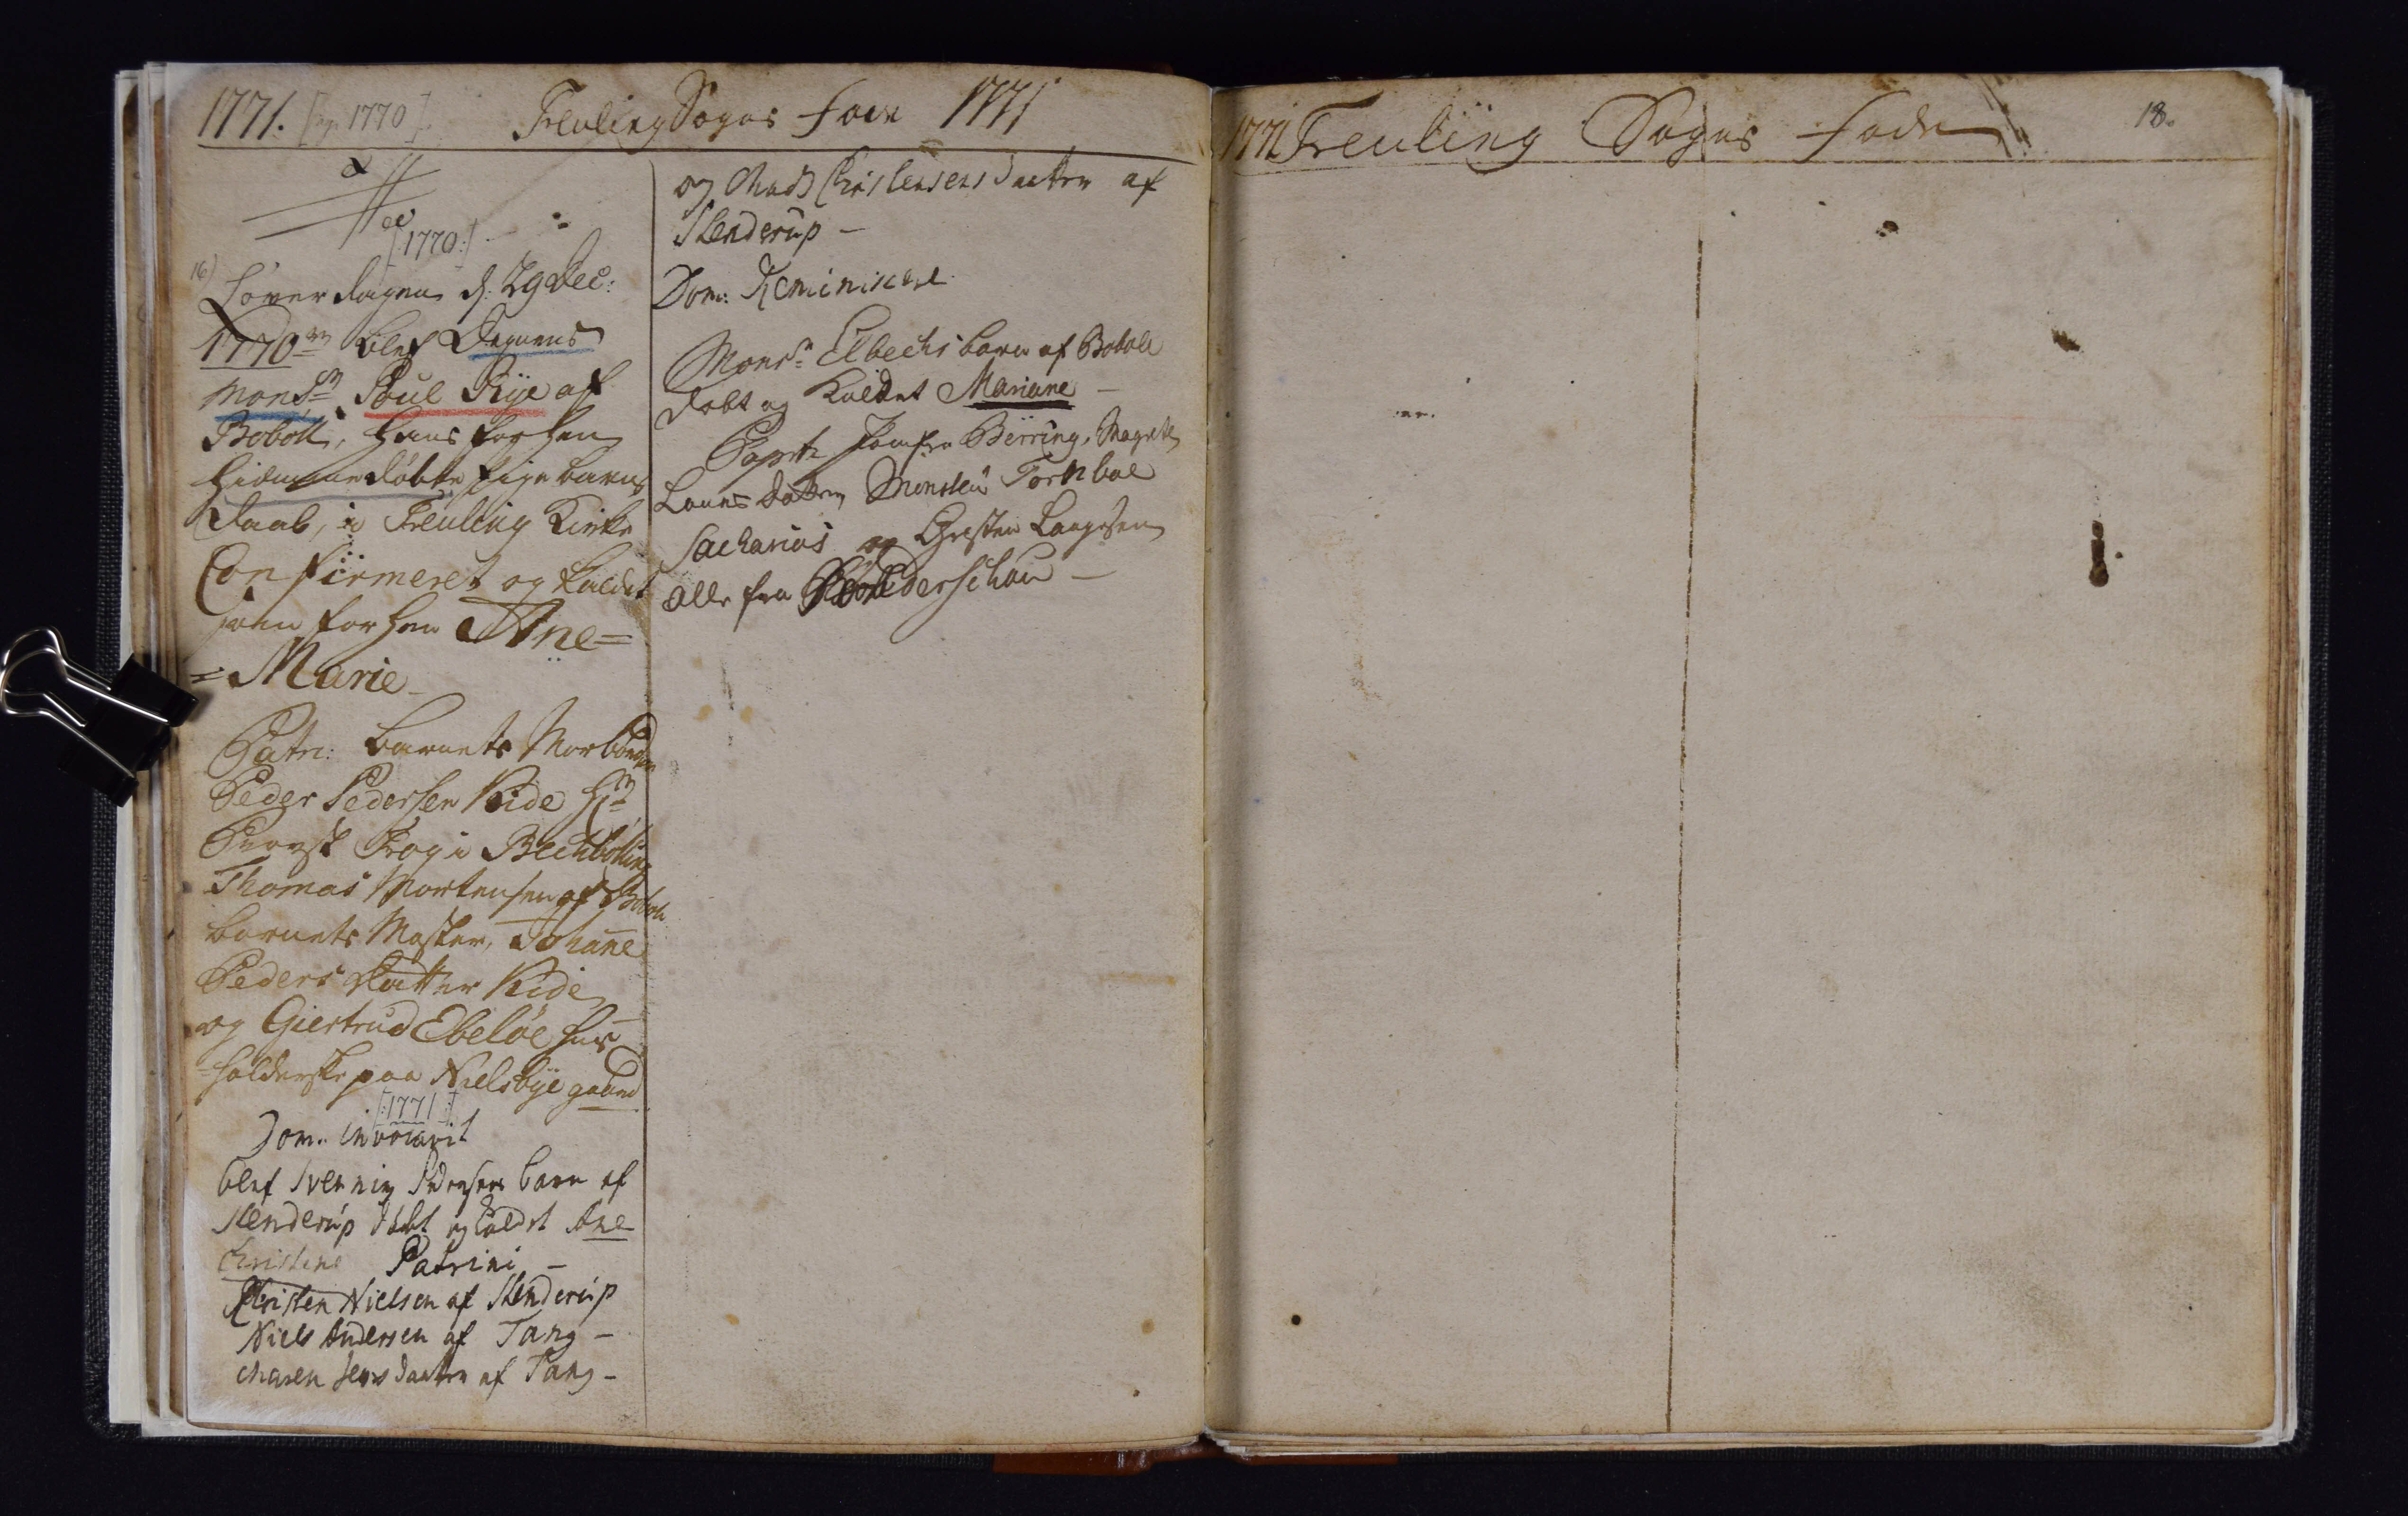1771. [og 1770].
Feuling Sogns Fode
[1770:]
16)
Løverdagen d: 29 Dec:
1770## blev Degnens
Monsr Poul Rye af
Bobøll, Hans forhen
Hiemmedøbte Pige Barns
Daab, i Feuling Kirsten
Confirmeret og kaldet
som forhen Ane =
= Marie
Patr: Barnets Morb##
Peder Pedersen Kide Hr
Provst Krog i Bechbølling
Thomas Mortensen af Boboll
Barnets Moster, Johanne
Peders Datter Kide.
og Giertrud Ebeløe Hus
= holderske paa Nielsbye gaard
[:1771:]
Dom. Invocavit
blef Svenning Pedersens barn af
Stenderup døbt og kaldet Ane
Christine Catrine
Christen Nielsen af Stenderup.
Niels Andersen af Tang -
Maren Jensdatter af Tang -
1771
og Madz Christensens Datter af
Stenderup
Dom. Reminiscere
Monsr Elbechs barn af Boboll
dobt og kaldet Mariane
Paptr Jomfr Berring, Magrete
Laues Datter M## Tor##
Sacharias og Christen Laugesen
alle fra ##derschou
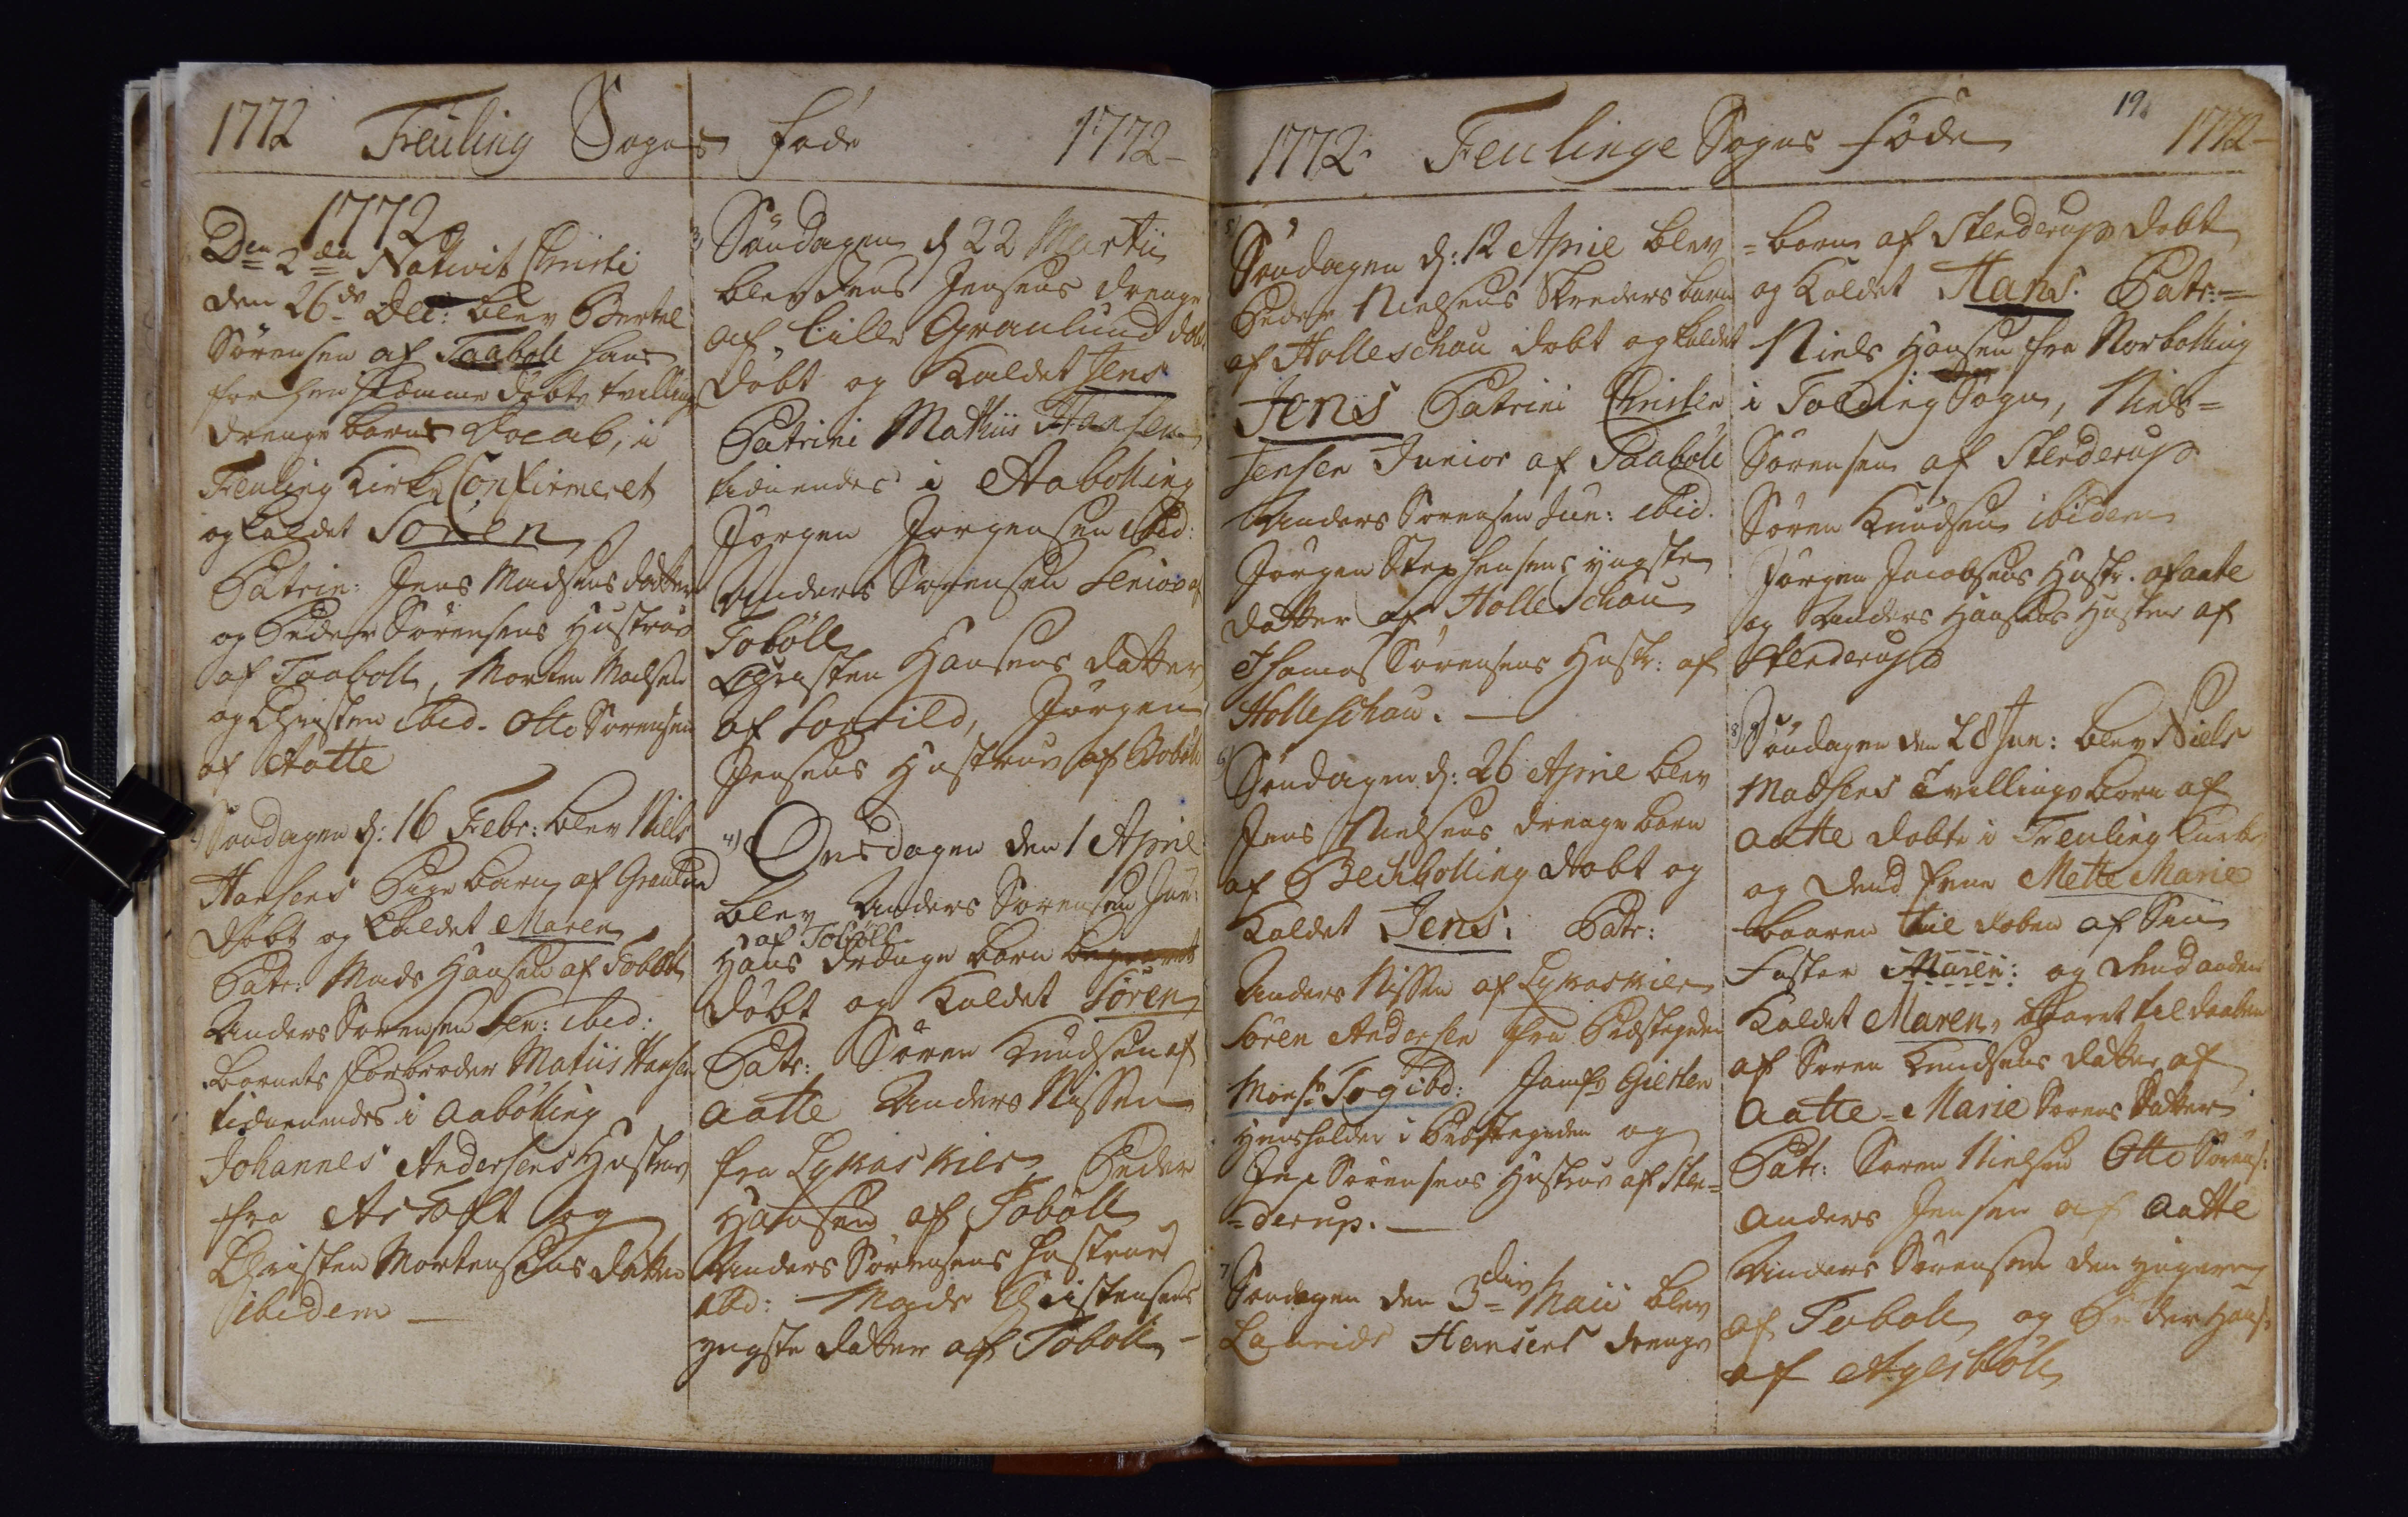1772 Feuling Sogns Føde
1772
Dca 2da Nativit Christi
den 26de Dec: blev Bertel
Sørensen af Taaboll hans
forhen Hæmme døbte tvilling
Drenge borns Daab, i
Feuling Kirke, Confirmeret
og kaldet Søren.
Patrin: Jens Madsens Datter
og Peder Sørensens Hustrue
af Taaboll, Morten Madsen
og Christen ibid. Otto Sorensen
af Aatte.
Sondagen d: 16 Febr: blev Niels
Hansens Pige Barn af Graulund
døbt og kaldet Maren
Patr: Mads Hansen af Toboll
Anders Sorensen Ser: ibid:
Barnets Forbroder Matiis Hansen
tiænnendes i Aabølling
Johannes Andersens Hustrue
fra Astoft og
Christen Mortensens datter
ibidem
1772
3,
Søndagen d 22 Martii
blev Jens Jensens Drenge
af Lille Graulund d##
døbt og kaldet Jens
Patrini Mathiis Hansen
tiænende i Aabolling
Jørgen Jørgensen ibid:
Anders Sørensen Senior af
Tobøll
Christen Hansens Datter
af Sorrild, Jørgen
Jensens Hustrue af Bobøll
4)
Onsdagen den 1 April
blev Anders Sørensen Jun
af Tobøll
Hans Drenge Barn begravet
døbt og kaldet Søren
Patr: Søren Knudsen af
Aatte Anders Nissen
fra Lykaskier Peder
Hansen af Tobøll
Anders Sørensens Hustrue
ibd: Mads Christensens
yngste Datter af Toboll
1772. Feulinge Sogns Føde
5)
Søndagen d: 12 April blev
Peder Nielsens Skreders barn
af Holleschou døbt og kaldet
Jens Patrini Christen
Jensen Junior af Taabøll
Anders Sørensen Jun: ibid:
Jørgen Stephansens yngste
Datter af Holleschou
Thomas Sørensens Hustr: af
Holleschou
6)
Søndagen d: 26 April blev
Jens Nielsens Drenge Barn
af Bechbolling dobt og
kaldet Jens: Patr:
Anders Nissen af Lykaskier
Søren Andersen fra Præsteg##den
Monsr Tog ibd: Jomfr Gierten
Huusholder i Præsteg##den og
Jep Sørensens Hustrue af Sten¬
= derup.
7
Søndagen den 3die Maii blev
Laurids Hansens drenge
19.
1772
= Barn af Stenderup døbt
og kaldet Hans. Patr: =
Niels Hansen fra Norbolling
i Folding Sogn, Niels =
Sørensen af Stenderup
Søren Knudsen ibidem
Jørgen Jacobsens Hustr. af Aatte
og Anders Hansens Hustrue af
Stenderup
8,
Søndagen den 28 Jun: blev Niels
Madsens Tvillinge barn af
Aatte døbte i Feuling Kirke
og dend Eene Mette Marie
baaren til Doben af Sin
Faster Maren og dend anden
kaldet Maren, baaret til Daaben
af Søren Knudsens Datter af
Aatte = Marie Sorens Datter
Patr: Soren Nielsøn Otto Sørens:
Anders Jensen af Aatte
Anders Sørensen den yngere
af Toboll og Peder Hans:
af Agesbøll